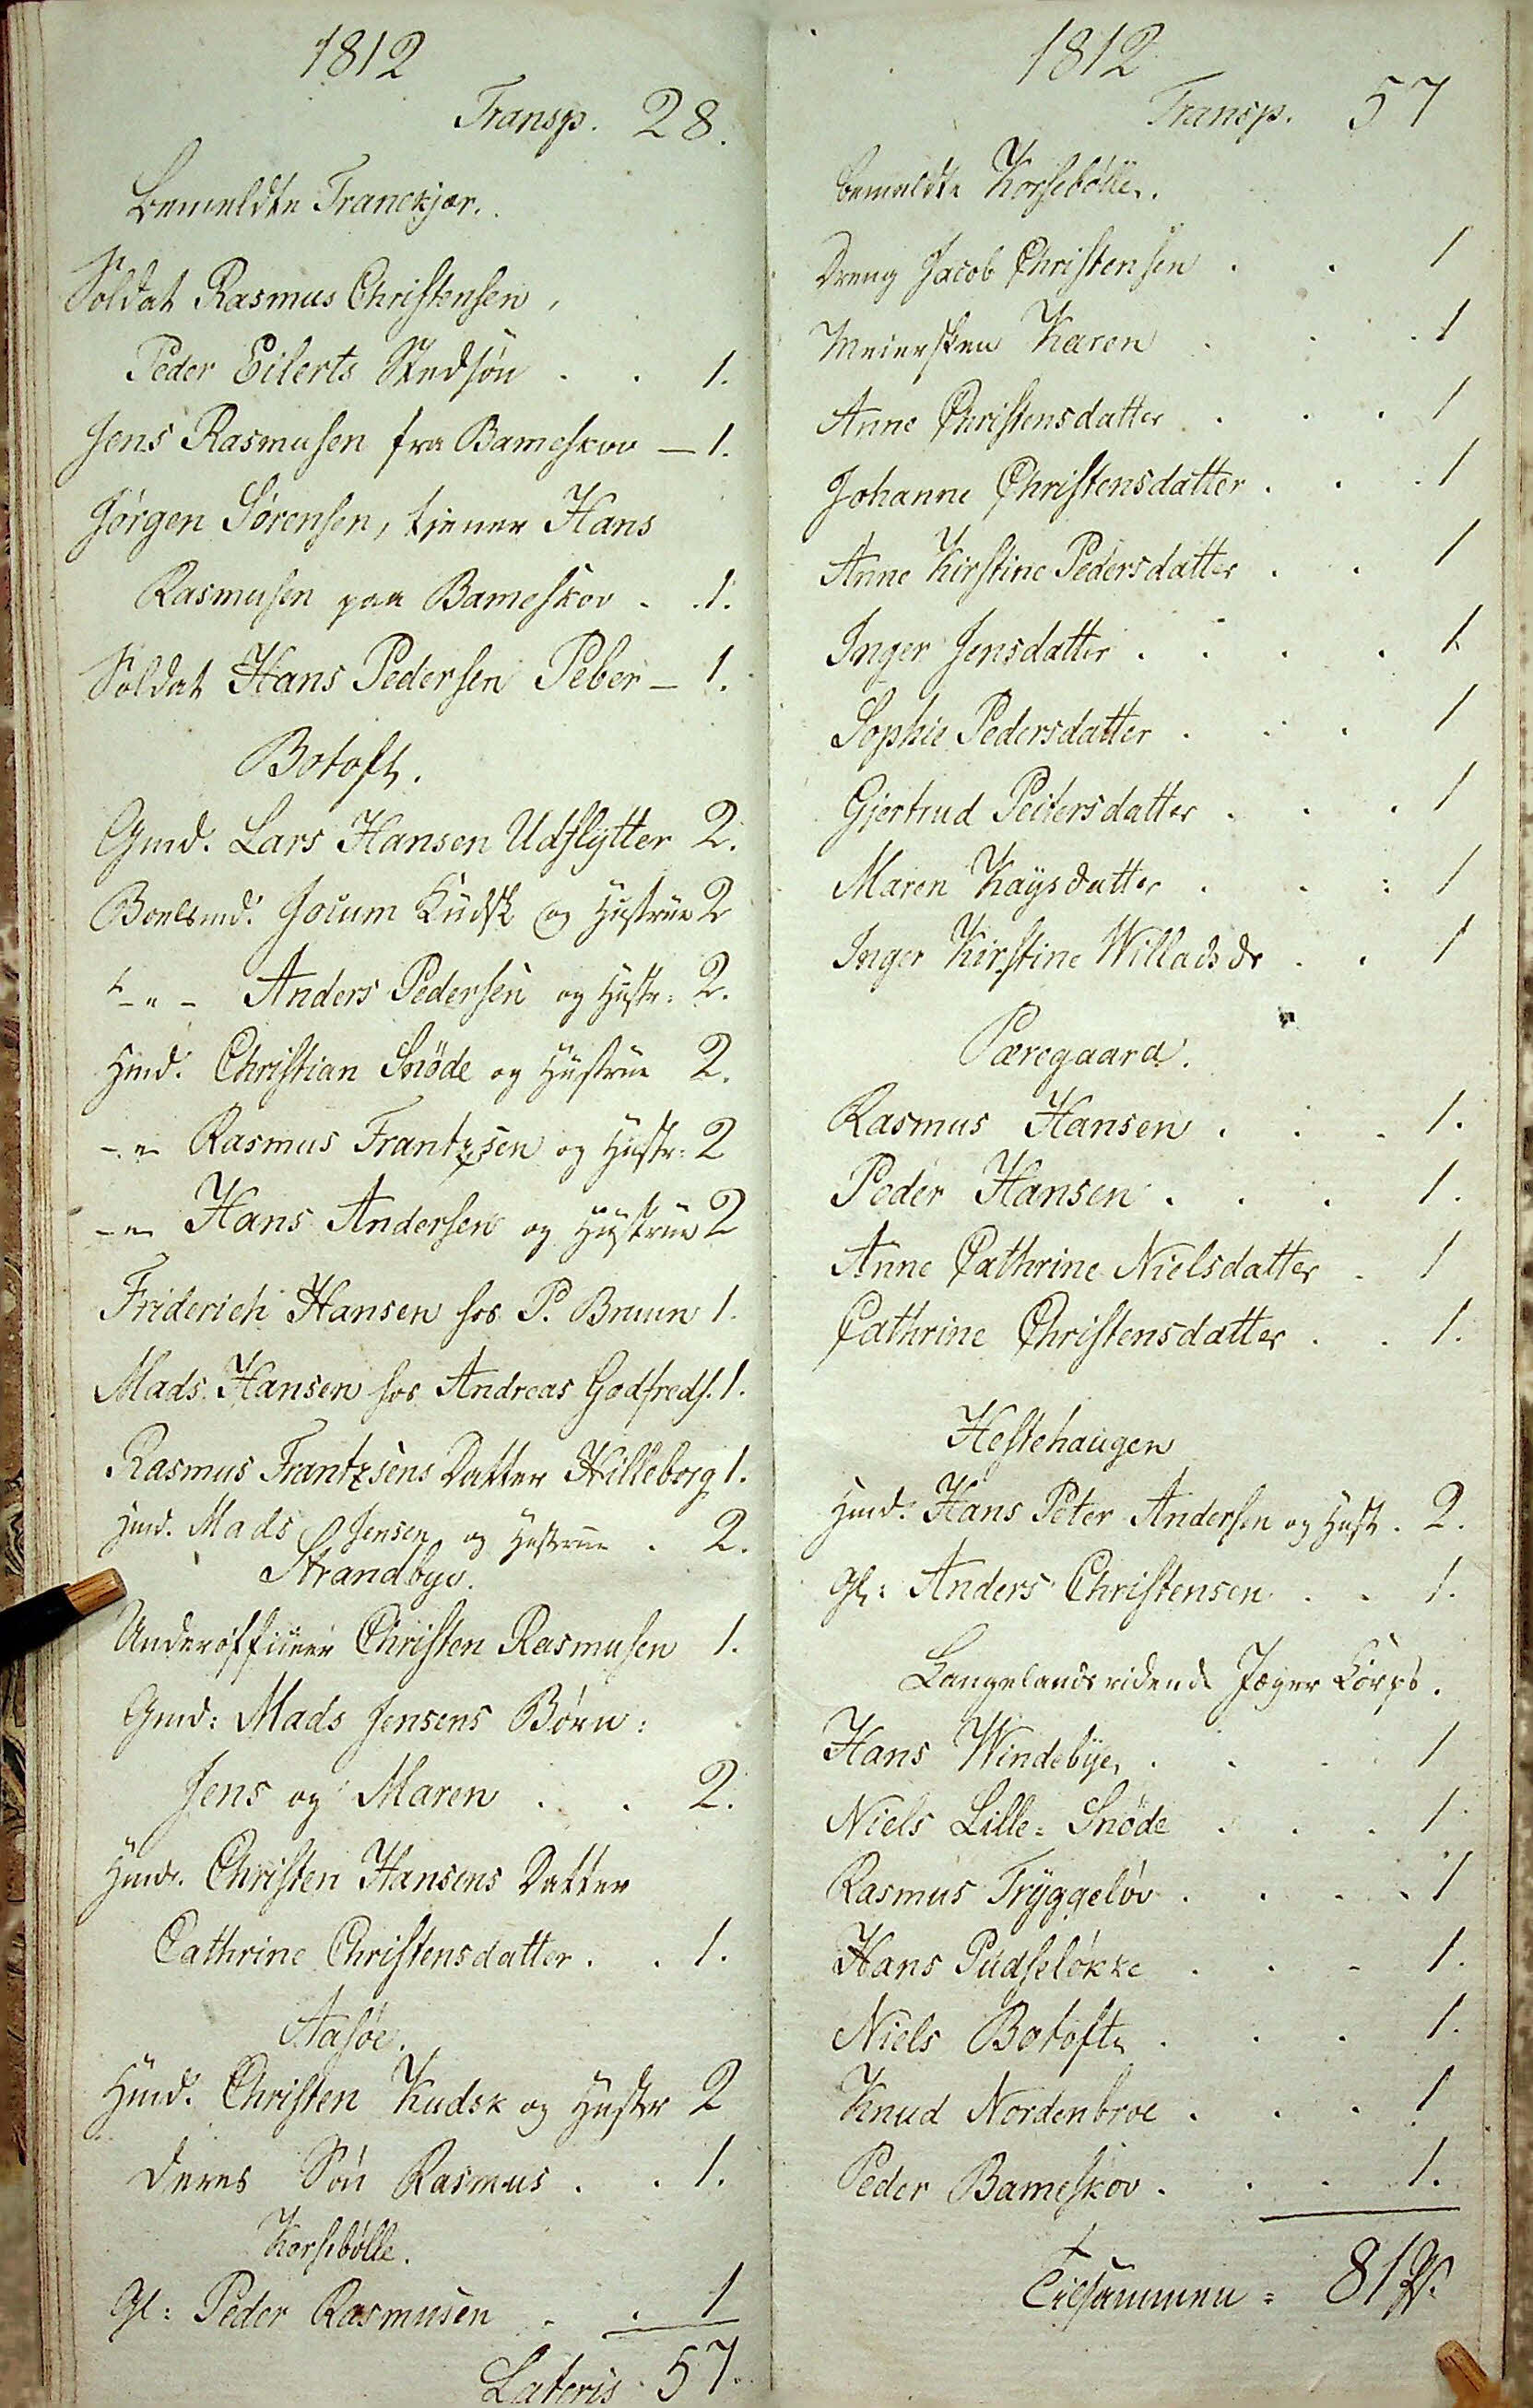1812
Transp. 28.
Bemmeldte Tranekjær.
Soldat Rasmus Christensen,
Peder Eilerts Stedsøn . . 1.
Jens Rasmusen fra Bameskov - 1.
Jørgen Sørensen, tjener Hans
Rasmusen paa Bameskov . . 1.
Soldat Hans Pedersen Peber - 1.
Botoft.
Gmd. Lars Hansen Udflytter 2.
Boelsmd. Jocum Kudsk og Hustrue 2
-"- Anders Pedersen og Hustr . 2.
Hmd. Christian Snøde og Hustrue 2.
-"- Rasmus Frantzsen og Hustr: 2
-"- Hans Andersen og Hustrue 2
Friderich Hansen hos P. Bruun 1.
Mads Hansen hos Andreas Godfreds . 1.
Rasmus Trantzsens Datter Hilleborg 1.
Hmd. Mads Jensen og Hustrue . 2.
Strandbye.
Underofficeer Christen Rasmusen 1.
Gmd: Mads Jensens Børn:
Jens og Maren . . 2.
Hmd. Christen Hansens Datter
Cathrine Christensdatter . . 1.
Aasøe.
Hmd. Christen Kudsk og Hustr 2
deres Søn Rasmus . . 1.
Korsebølle.
Gl: Peder Rasmusen . . 1
Lateris 57.
1812
Transp. 57
bemeldte Korsebølle.
Dreng Jacob Christensen . . 1
Meiersken Karen . . . 1
Anne Christensdatter . . . 1
Johanne Christensdatter . . . 1
Anne Kirstine Pedersdatter . . 1
Jnger Jensdatter . . . . 1
Sophie Pedersdatter . . . 1
Giertrud Peitersdatter . . . 1
Maren Kaysdatter . . : 1
Inger Kirstine Willadsdr . . 1
Pæregaard.
Rasmus Hansen . . . 1.
Peder Hansen . . . 1
Anne Cathrine Nielsdatter . 1
Cathrine Christensdatter . . 1.
Hestehaugen
Hmd. Hans Peter Andersen og Hust . 2.
Gl: Anders Christensen . . 1.
Langelands ridende Jæger Corps
Hans Windebye . . . . 1
Niels Lille = Snøde . . . 1
Rasmus Tryggeløv . . . . 1
Hans Pudseløkke . . . 1
Niels Botofte . . . 1
Knud Nordenbroe . . . 1
Peder Bameskov . . . 1.
Tilsammen: 81 Ps.
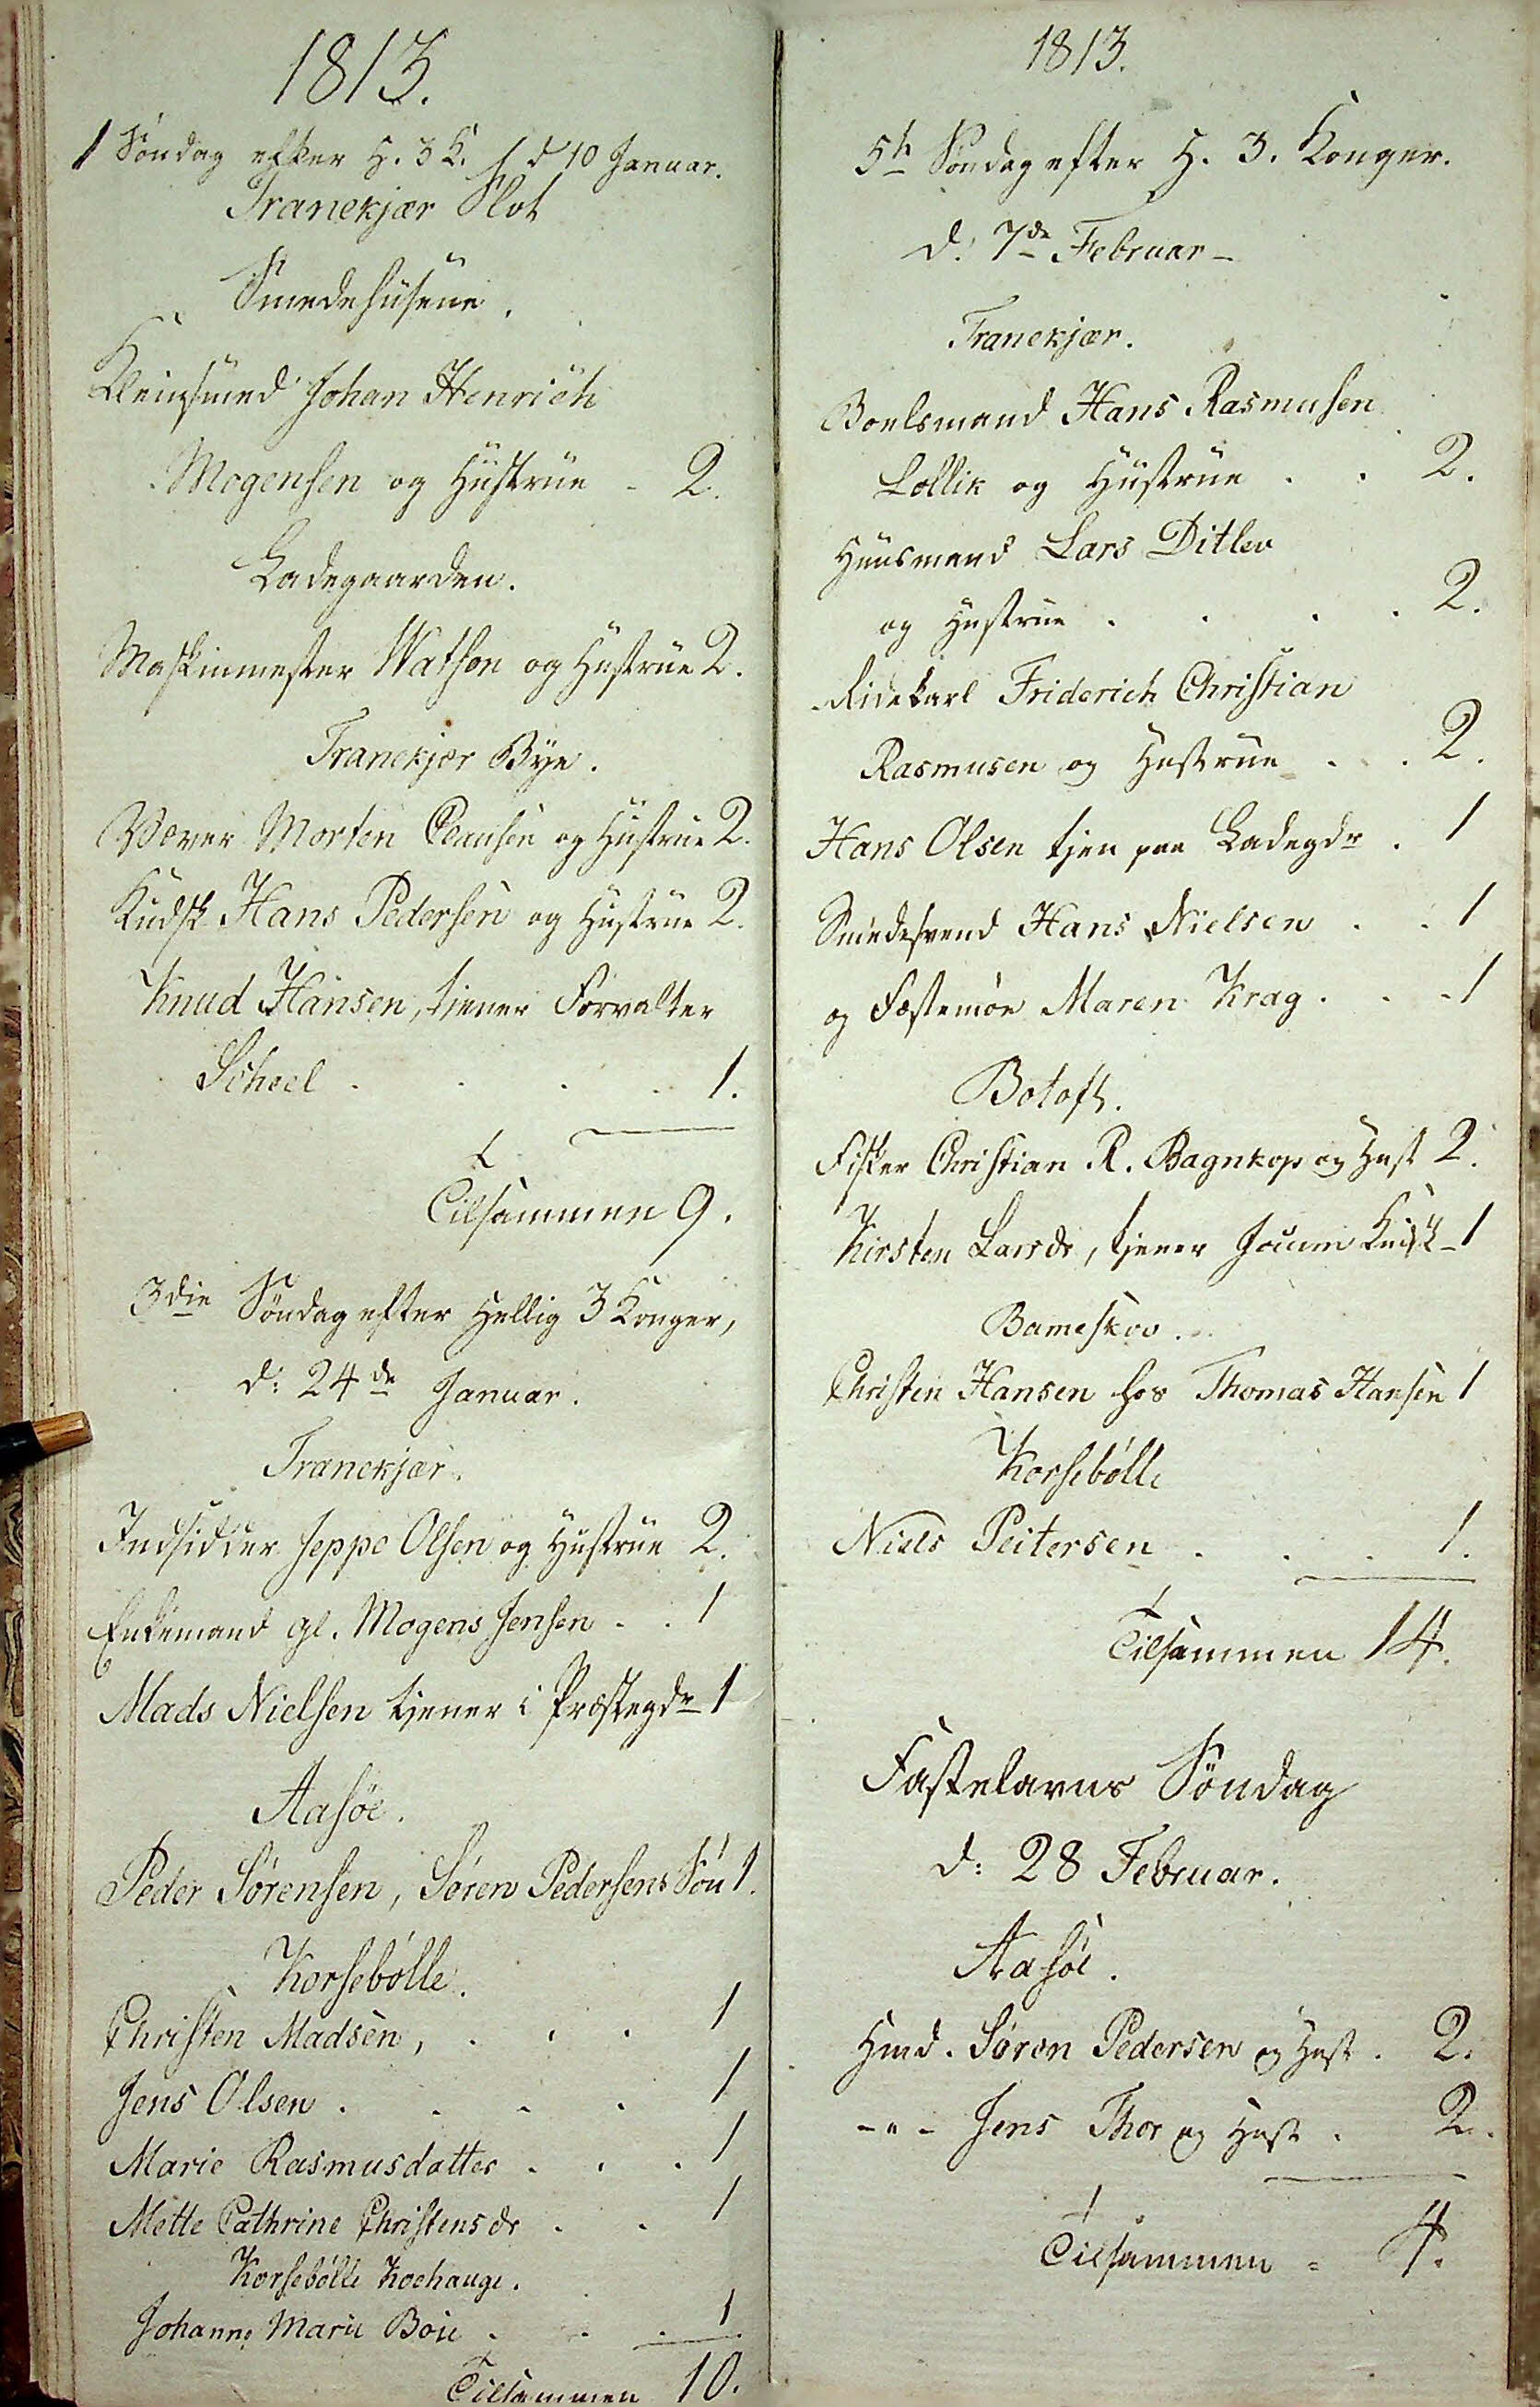1813.
1 Søndag efter H. 3 K. d 10 Januar.
Tranekjær Slot
Smedehusene
Kleinsmed Johan Henrich
Mogensen og Hustrue - 2.
Ladegaarden.
Maskinmester Watson og Hustrue 2.
Tranekjær Bye.
Væver Morten Clausen og Hustrue 2.
Kudsk Hans Pedersen og Hustrue 2.
Knud Hansen, tjener Forvalter
Scheel . . . . 1.
Tilsamming 9.
3die Søndag efter Hellig 3 Konger,
d: 24de Januari
Tranekjær.
Indsidder Jeppe Olsen og Hustrue 2.
Enkemand gl. Mogens Jensøn . .1
Mads Nielsen tjener i Præstegdr 1
Aasøe.
Peder Sørensen, Sørens Pedersens Søn 1.
Korsebølle.
Christen Madsen . . . . 1
Jens Olsen . . . . 1
Marie Rasmusdatter . . . 1
Mette Cathrine Christensdr . . 1
Korsebølle Koehauge.
Johanne Marie Boie . . . 1
Tilsamme 10. 
1813.
5te Søndag efter H. 3. Konger.
d: 7de Februar-
Tranekjær
Boelsmand Hans Rasmusen
Lollik og Hustrue . . 2.
Huusmand Lars Ditlev
og Hustrue . . . . 2.
Ridekarl Friderich Christian
Rasmusen og Hustrue . .2
Hans Olsen tjen paa Ladegdr . 1
Smedesvend Hans Nielsen . . 1
og Fæstemøe Maren Krag . . . 1
Botoft
Fisker Christian R. Bagnkop og Hust 2.
Kirsten Larsdr, tjener Jocum Kudsk## - 1
Bameskov.
Christen Hansen hos Thomas Hansen 1
Korsebølle
Niels Peitersen . . . 1.
Tilsammen 14.
Fastelavns Søndag
d: 28 Februar.
Aasøe
Hmd. Søren Pedersen og Hust . 2.
-"- Jens Thor og Hust: 2.
Tilsammen= 4.